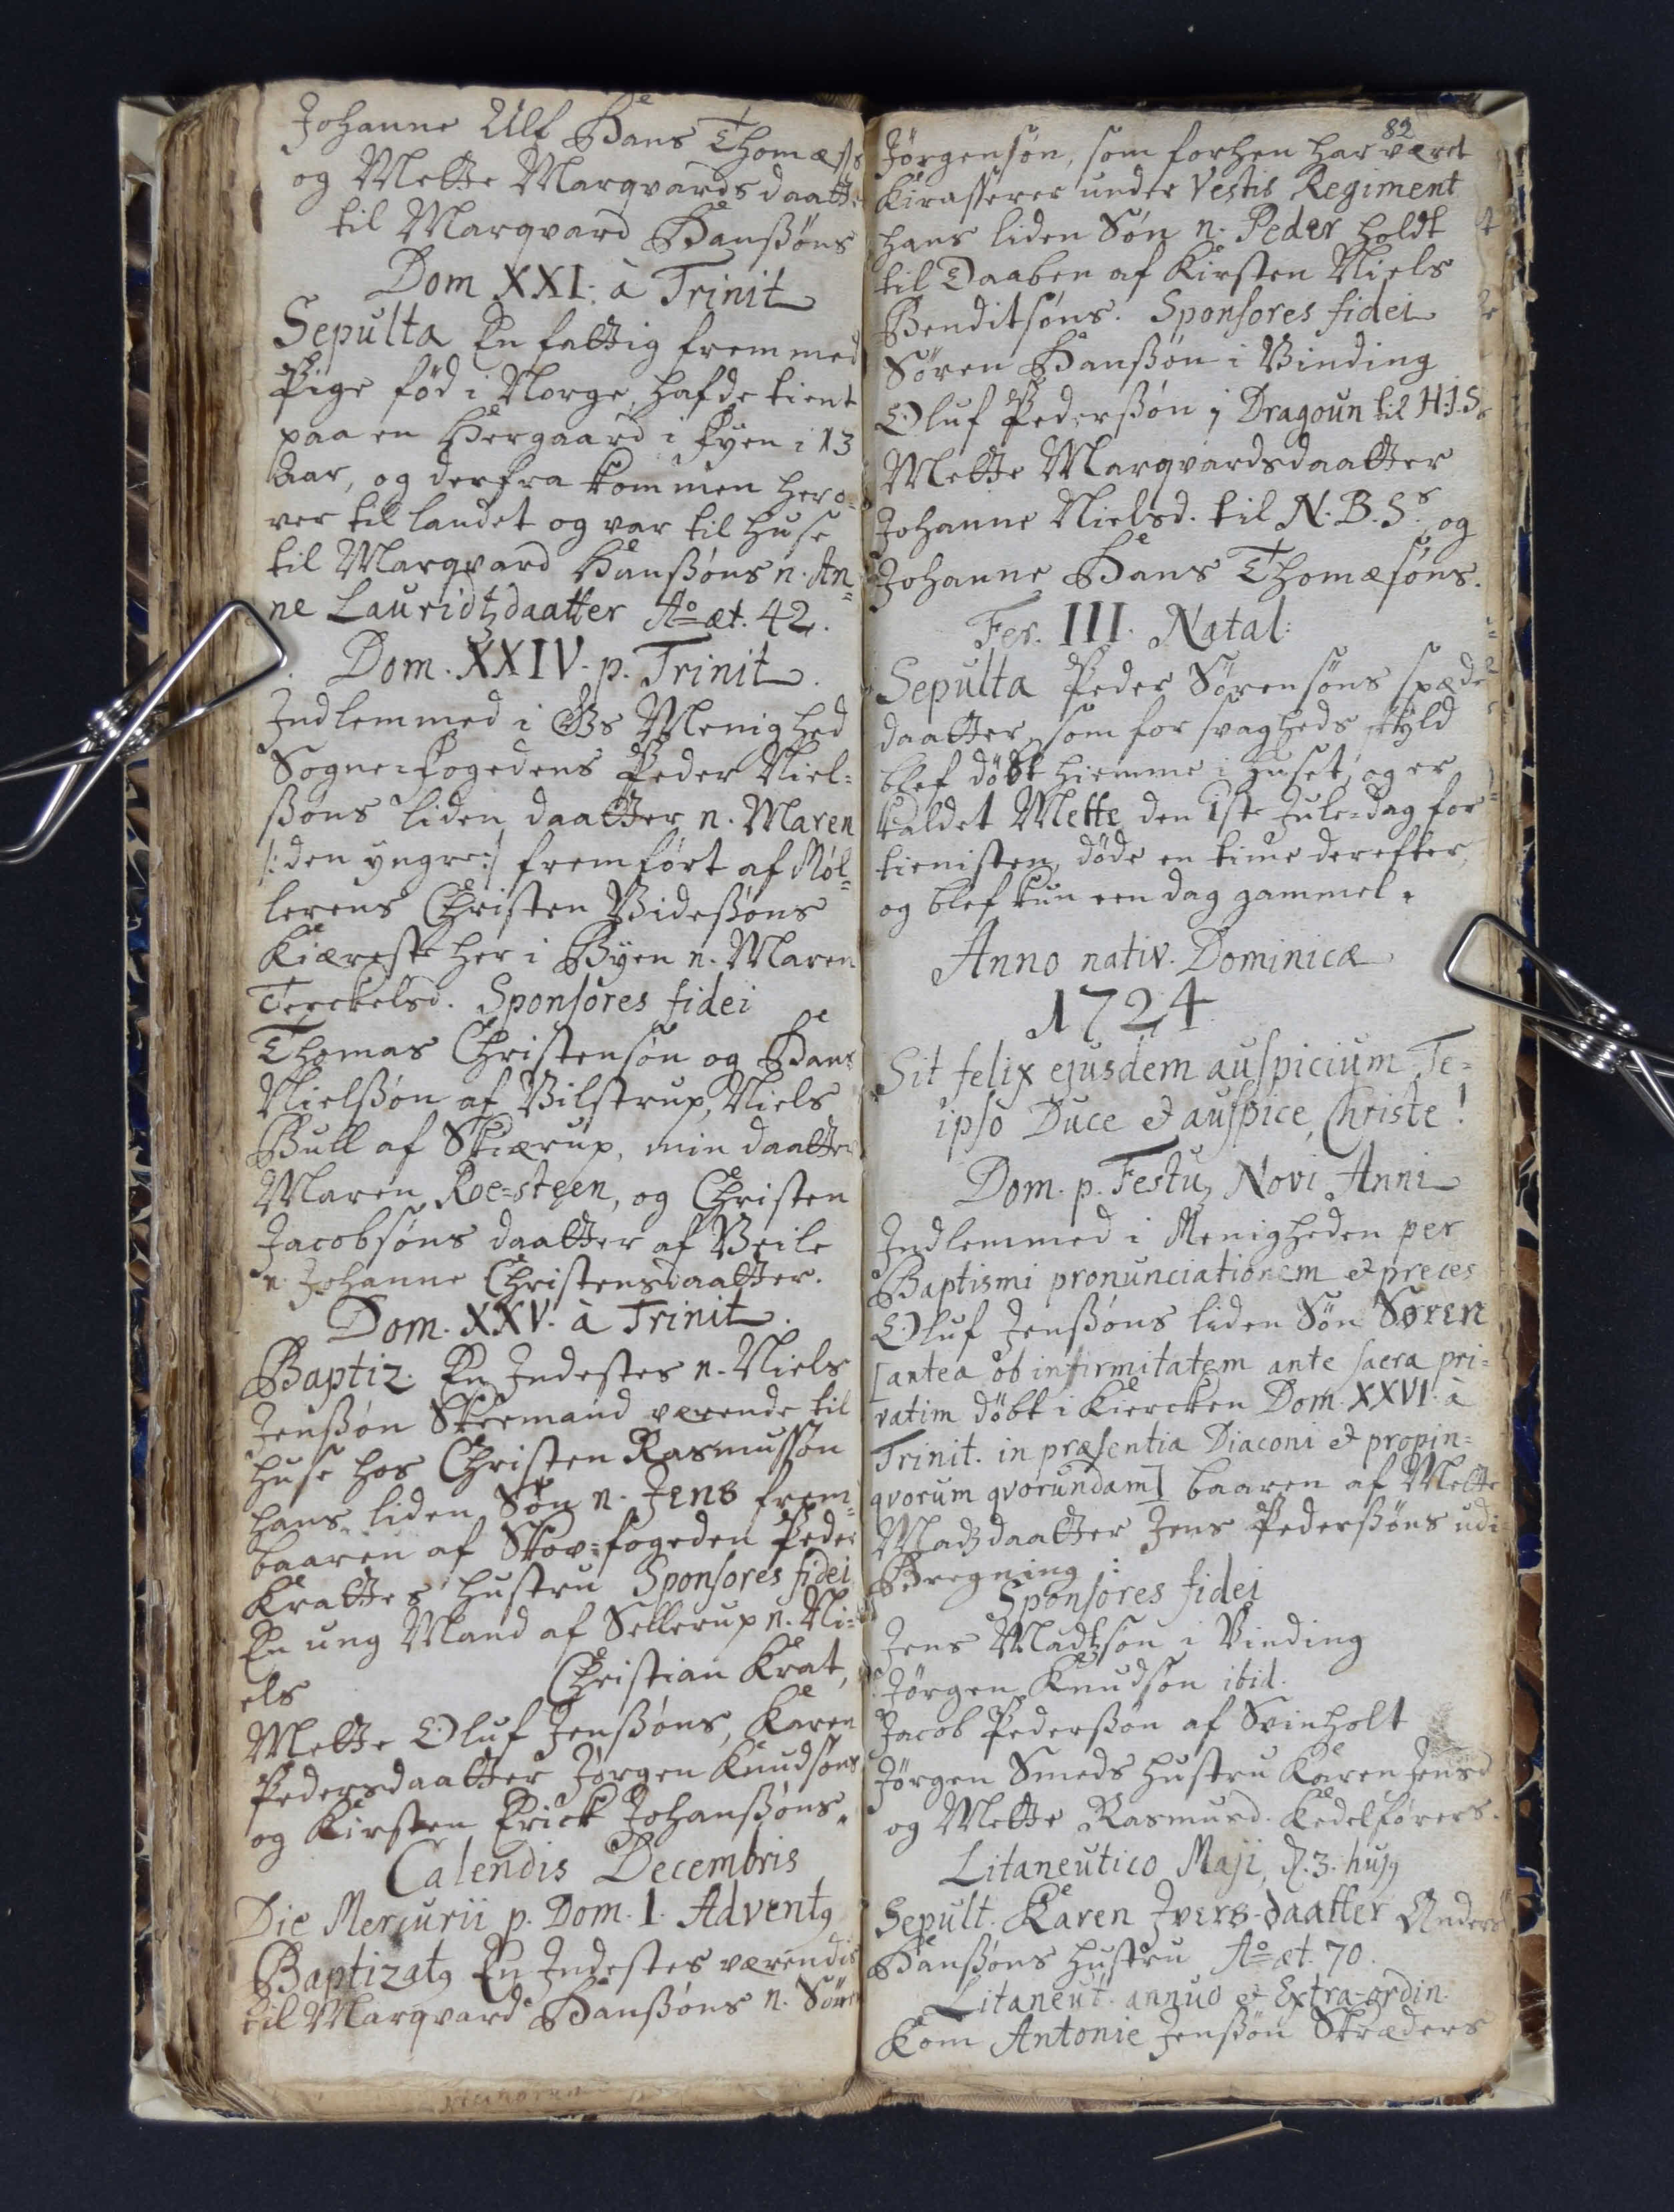Johanne Ulf Hans Thomæss
og Mette Marqvardsdaatt 
til Marqvard Hanssøns
Dom. XXI: a Trinit
Sepulta En fattig fremmed
Pige fød i Norge, hafde tient 
paa en Hergaard i Fyen i 13 
Aar, og derfra kom men her o¬
ver til landet og var til huse
til Marqvard Hanssøns n. An¬
ne Lauridtzdaatter Ao æt. 42.
Dom. XXIV. p. Trinit
Indlemmed i Gs Menighed
Sogne=fogedens Peder Niel¬
ssøns liden daatter n. Maren
/: den yngre :/ fremført af Møl¬
lerens Christen Videssøns
Kiæreste her i Byen n. Maren
Terckelsd. Sponsoren fidei
Thomas Christensøn og Hans
Nielssøn af Vilstrup, Niels
Bull af Skiærup, min daatter
Maren Roe=steen, og Christen
Jacobsøns daatter af Veile
n. Johanne Christensdaatter.
Dom. XXV. a Trinit.
Baptiz. En Indestes n. Niels
Jenssøn Skeemand værende til
huse hos Christen Rasmussøn
hans liden Søn n. Jens frem¬
baaren af Skov=fogeden Peder
Krattes hustru Sponsores fidei
En ung Mand af Sellerup n. Ni¬
els. 
Christian Krat,
Mette Oluf Jenssøns, Karen
Pedersdaatter Jørgen Knudsøns
og Kirsten Erick Johanssøns.
Calendis Decembris 
Die Mercurii p. Dom. 1 Adventq
Baptizatq En Indestes værendis
til Marqvard Hanssøns n. Søren
82
Jørgensøn, som forhen har været
Kirasserer under Vestis Regiment
hans liden Søn n. Peder holdt
til Daaben af Kirsten Niels
Benditsøns. Sponsores fidei
Søren Hanssøn i Vinding
Oluf Pederssøn 1 Dragoun til H.J.S
Mette Marqvardsdaatter
Johanne Nielsd. til N.B.Ss. og
Johanne Hans Thomæsøns.
Fer. III. Natal:
Sepulta Peder Sørensøns spæde
daatter som for svagheds skyld
blef døbt hiemme i huset, og er
kaldet Mette den 1ste Jule=dag for
tienisten, døde en time derefter,
og blef kun een dag gammel.
Anno nativ. Dominicæ
1724.
Sit felix ejusdem aupicium Te¬
ipso Duce et auspice, Christe!
Dom. p. Festuz Novi Anni
Indlemmed i Menigheden per
Baptismi pronunciationem et preces
Oluf Jenssøns liden Søn Søren
[antea ob infirmitatem ante saera pri¬
vatim døbt i Kiercken Dom. XXVI. a
Trinit. in præsentia Diaconi et propin¬
qvorum qvorundam] baaren af Mette
Madtzdaatter Jens Pederssøns udi
Breining.
Sponsores fidei
Jens Madtzen i Vinding
Jørgen Knudsøn ibid.
Jacob Pederssøn af Svinholt
Jørgen Smeds hustru Karen Jensd
og Mette Rasmusd. Kedelførers
Litaneutico Maji d. 3. hujq
Sepult. Karen Ivers-daatter Anders
Hanssøns hustru Ao æt. 70
Litaneut. annuo et Extra-ordin.
Kom Antonie Jenssøn Skræders
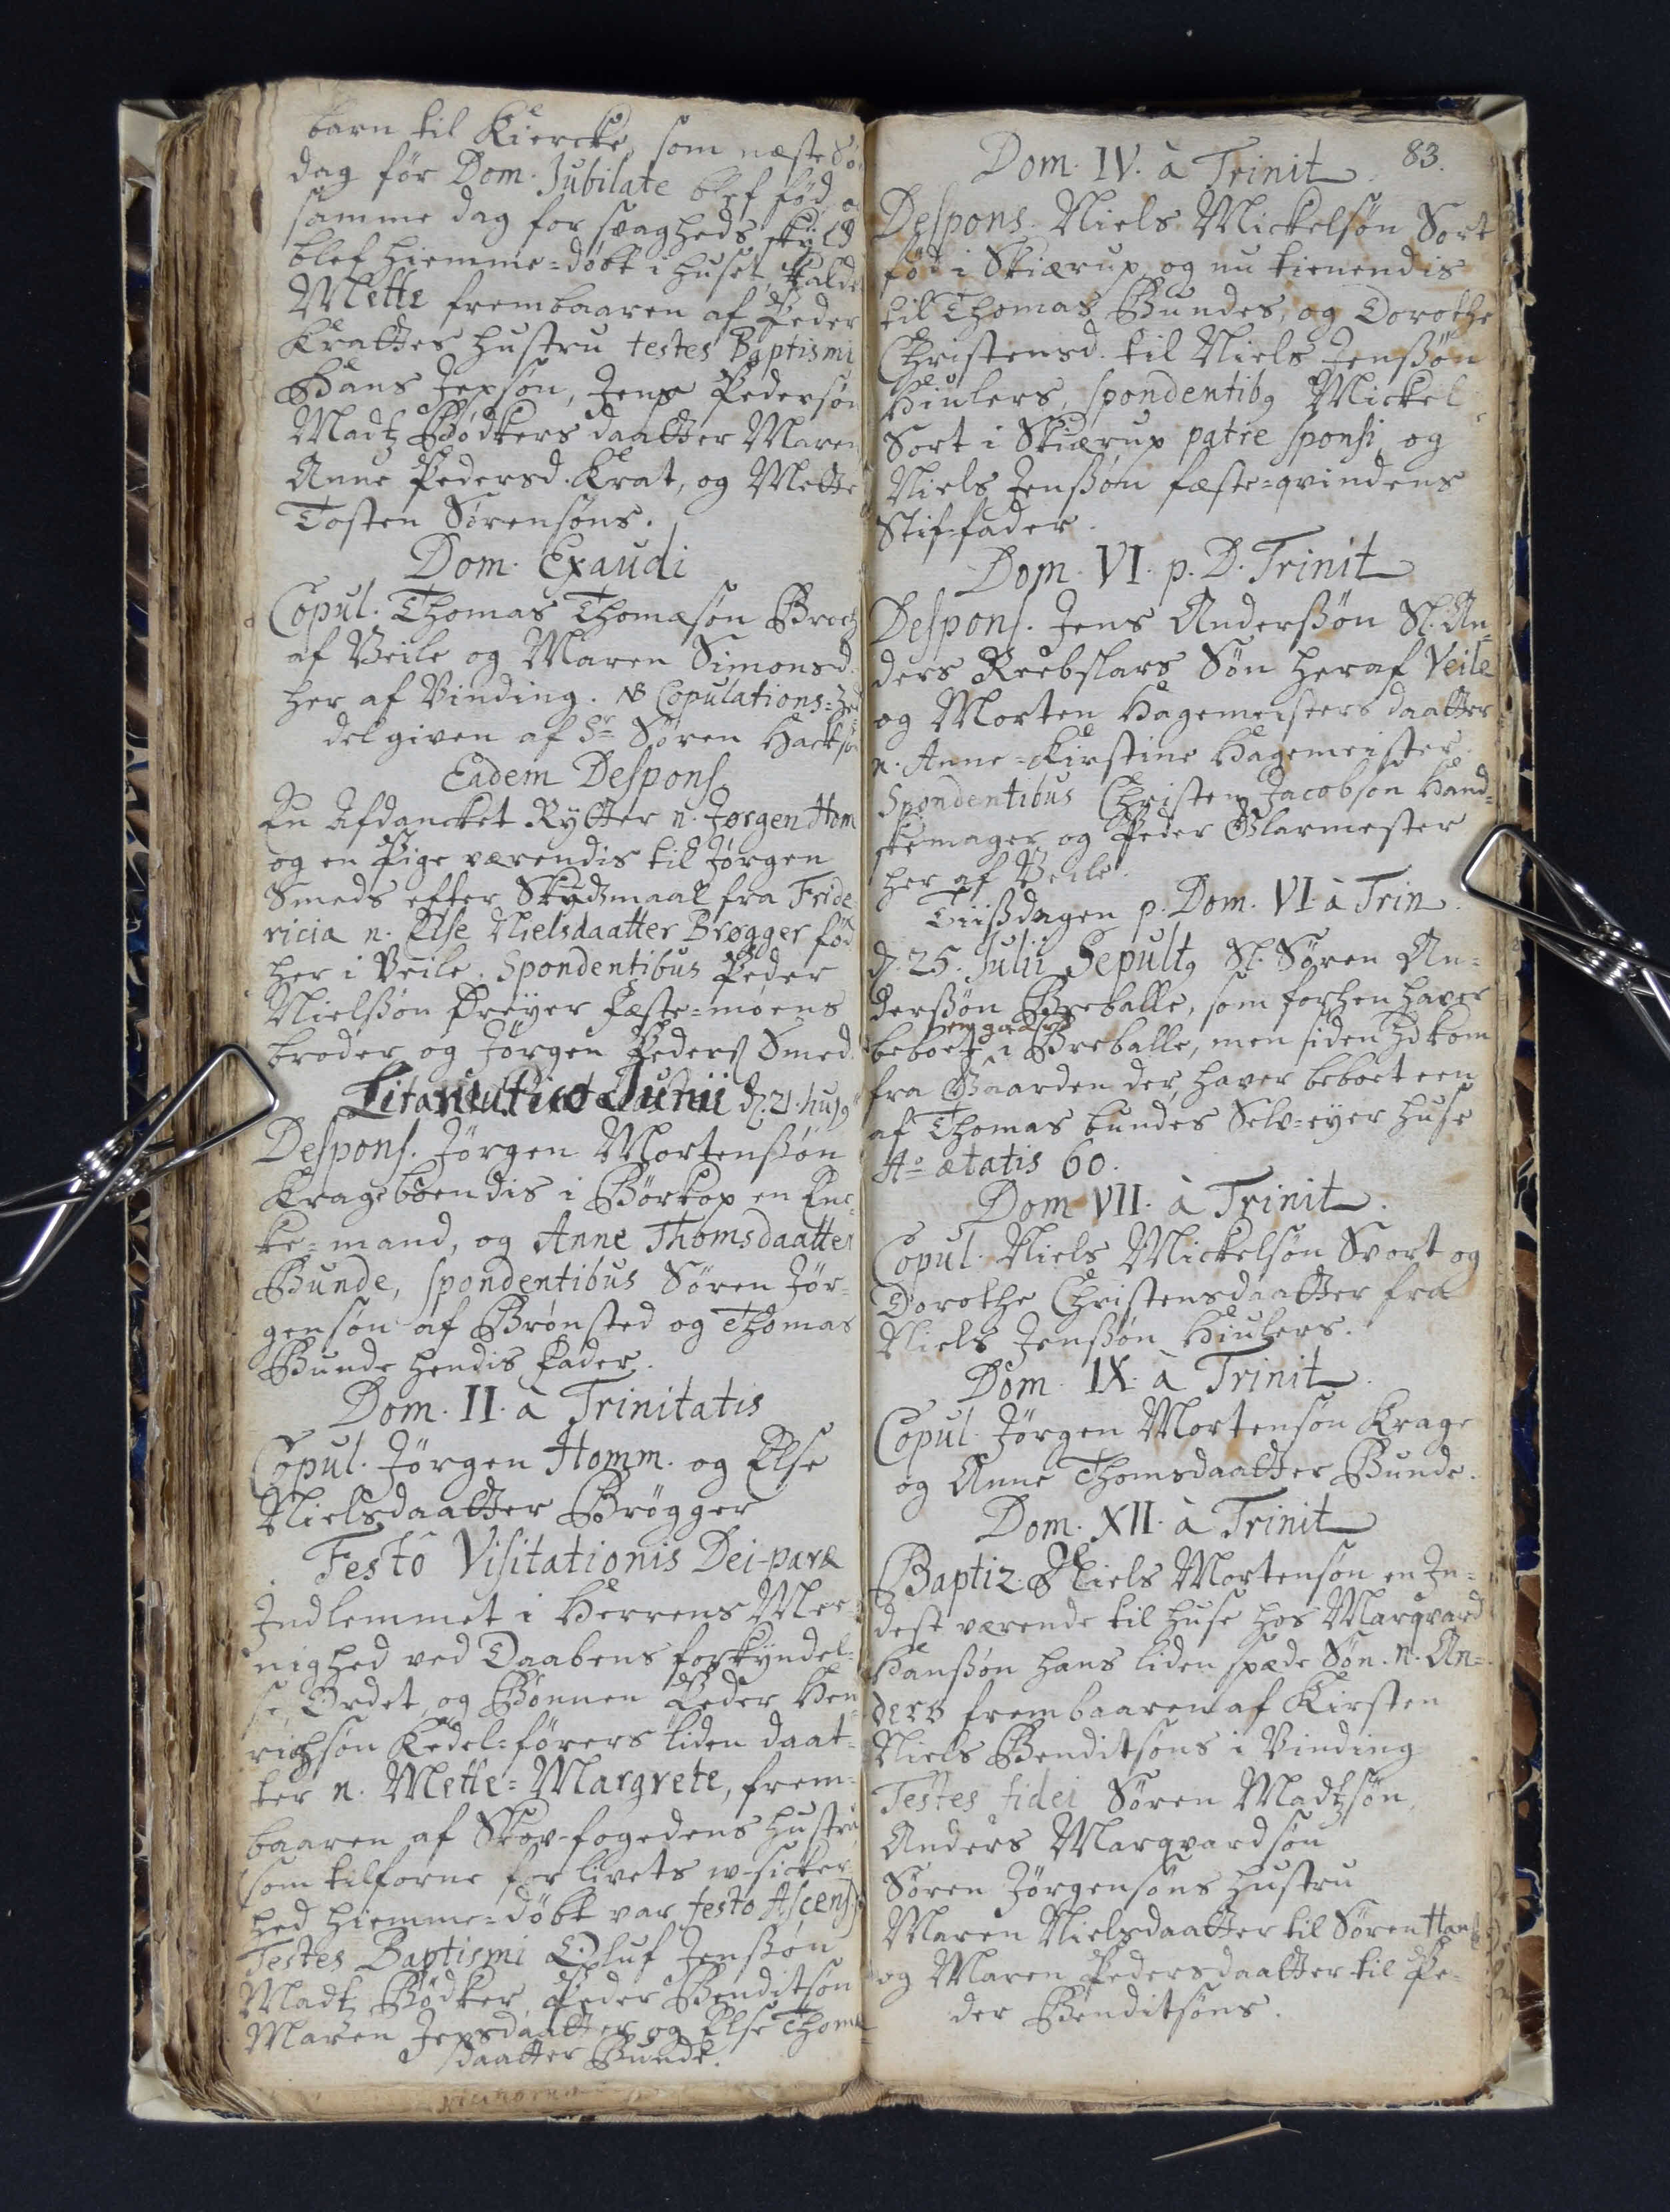barn til Kiercke, som næste Søn
dag før Dom. Jubilate blef fød og
samme dag for svagheds skyld
blef hiemme=døbt i huset, kaldet 
Mette frembaaren af Peder
Krattes hustru testes Baptismi
Hans Jepsøn, Jens Pedersøn
Madtz Bødkers daatter Maren
Anne Pedersd. Krat, og Mette
Tosten Sørensøn.
Dom. Exaudi
Copul. Thomas Thomæsøn Broch
af Veile og Maren Simonsd
her af Vinding. NB. Copulations=Zed¬
del given af Sr Søren Hackso 
Eadem Despons.
En Afdancket Rytter n. Jørgen Hom
og en Pige værendis til Jørgen
Smeds efter Skudzmaal fra Fride¬
ricia n. Else Nielsdaatter Brøgger, fød
her i Veile. Spondentibus Peder
Nielssøn Dreyer Fæste=møens
broder og Jørgen Peders Smed.
Liean##tico Junii d. 21 hujq
Despons. Jørgen Mortenssøn
Krage boendis i Børkop en Enc¬
ke=mand, og Anne Thomasdaatter
Bunde, spondentibus Søren Jør¬
gensøn af Brønsted og Thomas
Bunde hendis Fader
Dom. II. a Trinitatis
Copul. Jørgen Homm. og Else
Nielsdaatter Brøgger
Festo Visitationis Dei-paræ
Indlemmet i Herrens Mee¬
nighed ved Daabens forkyndel¬
se, Ordet, og Bønnen Peder Hen¬
richsøn Kedel=førers liden daat¬
ter n. Mette=Margrete, frem¬
baaren af Skov-fogedens hustru
(som tilforne for livets w-sicker¬
hed hiemme=døbt var festo Ascens.)
Testes Baptismi Oluf Jenssøn
Madtz Bødker, Peder Benditsøn
Maren Jepsdaatter og Else Thom 
daatter Bunde.
83.
Dom. IV. a Trinit
Despons. Niels Mickelsøn Sort
fød i Skiærup, og nu tienendis 
til Thomas Bundes, og Dorothe
Christensd. til Niels Jenssøn
Hiulers, Spondentibq Mickel
Sort i Skiærup patre sponsi og
Niels Jenssøn fæste=qvindens
Stif=fader
Dom. VI. a D. Trinit
Despons. Jens Anderssøn Sl. An¬
ders Reebslars Søn heraf Veile
og Morten Hagemeisters daatter
n. Anne=Kirstine Hagemeister
Spondentibus Christen Jacobsøn Hand¬
skemager og Peder Glarmester
her af Veile
Tiissdagen p. Dom. VI. a Trin
d. 25. Julii Sepultq Sl. Søren An¬
derssøn Breballe, som forhen haver
en grd##
beboet i Breballe, men siden hd kom
fra Gaarden der, haver beboet een
af Thomas Bundes Selv=eyer huse
Ao ætatis 60
Dom. VII. a Trinit
Copul. Niels Mickelsøn Svort og
Dorothe Christensdaatter fra 
Niels Jenssøn Hiulers.
Dom. IX. a Trinit
Copul. Jørgen Mortensøn Krage
og Anne Thomasdaatter Bunde.
Dom. XII. a Trinit
Baptiz. Niels Mortensøn en In
dest værende til huse hos Marqvard 
Hanssøn hans liden spæde Søn. n. An¬
ders frembaaren af Kirsten
Niels Benditsøns i Vinding
Testes fidei Søren Madtzsøn
Anders Marqvardsøn
Søren Jørgensøns hustru
Maren Nielsdaatter til Søren Hans
og Maren Pedersdaatter til Pe¬
der Benditsøns